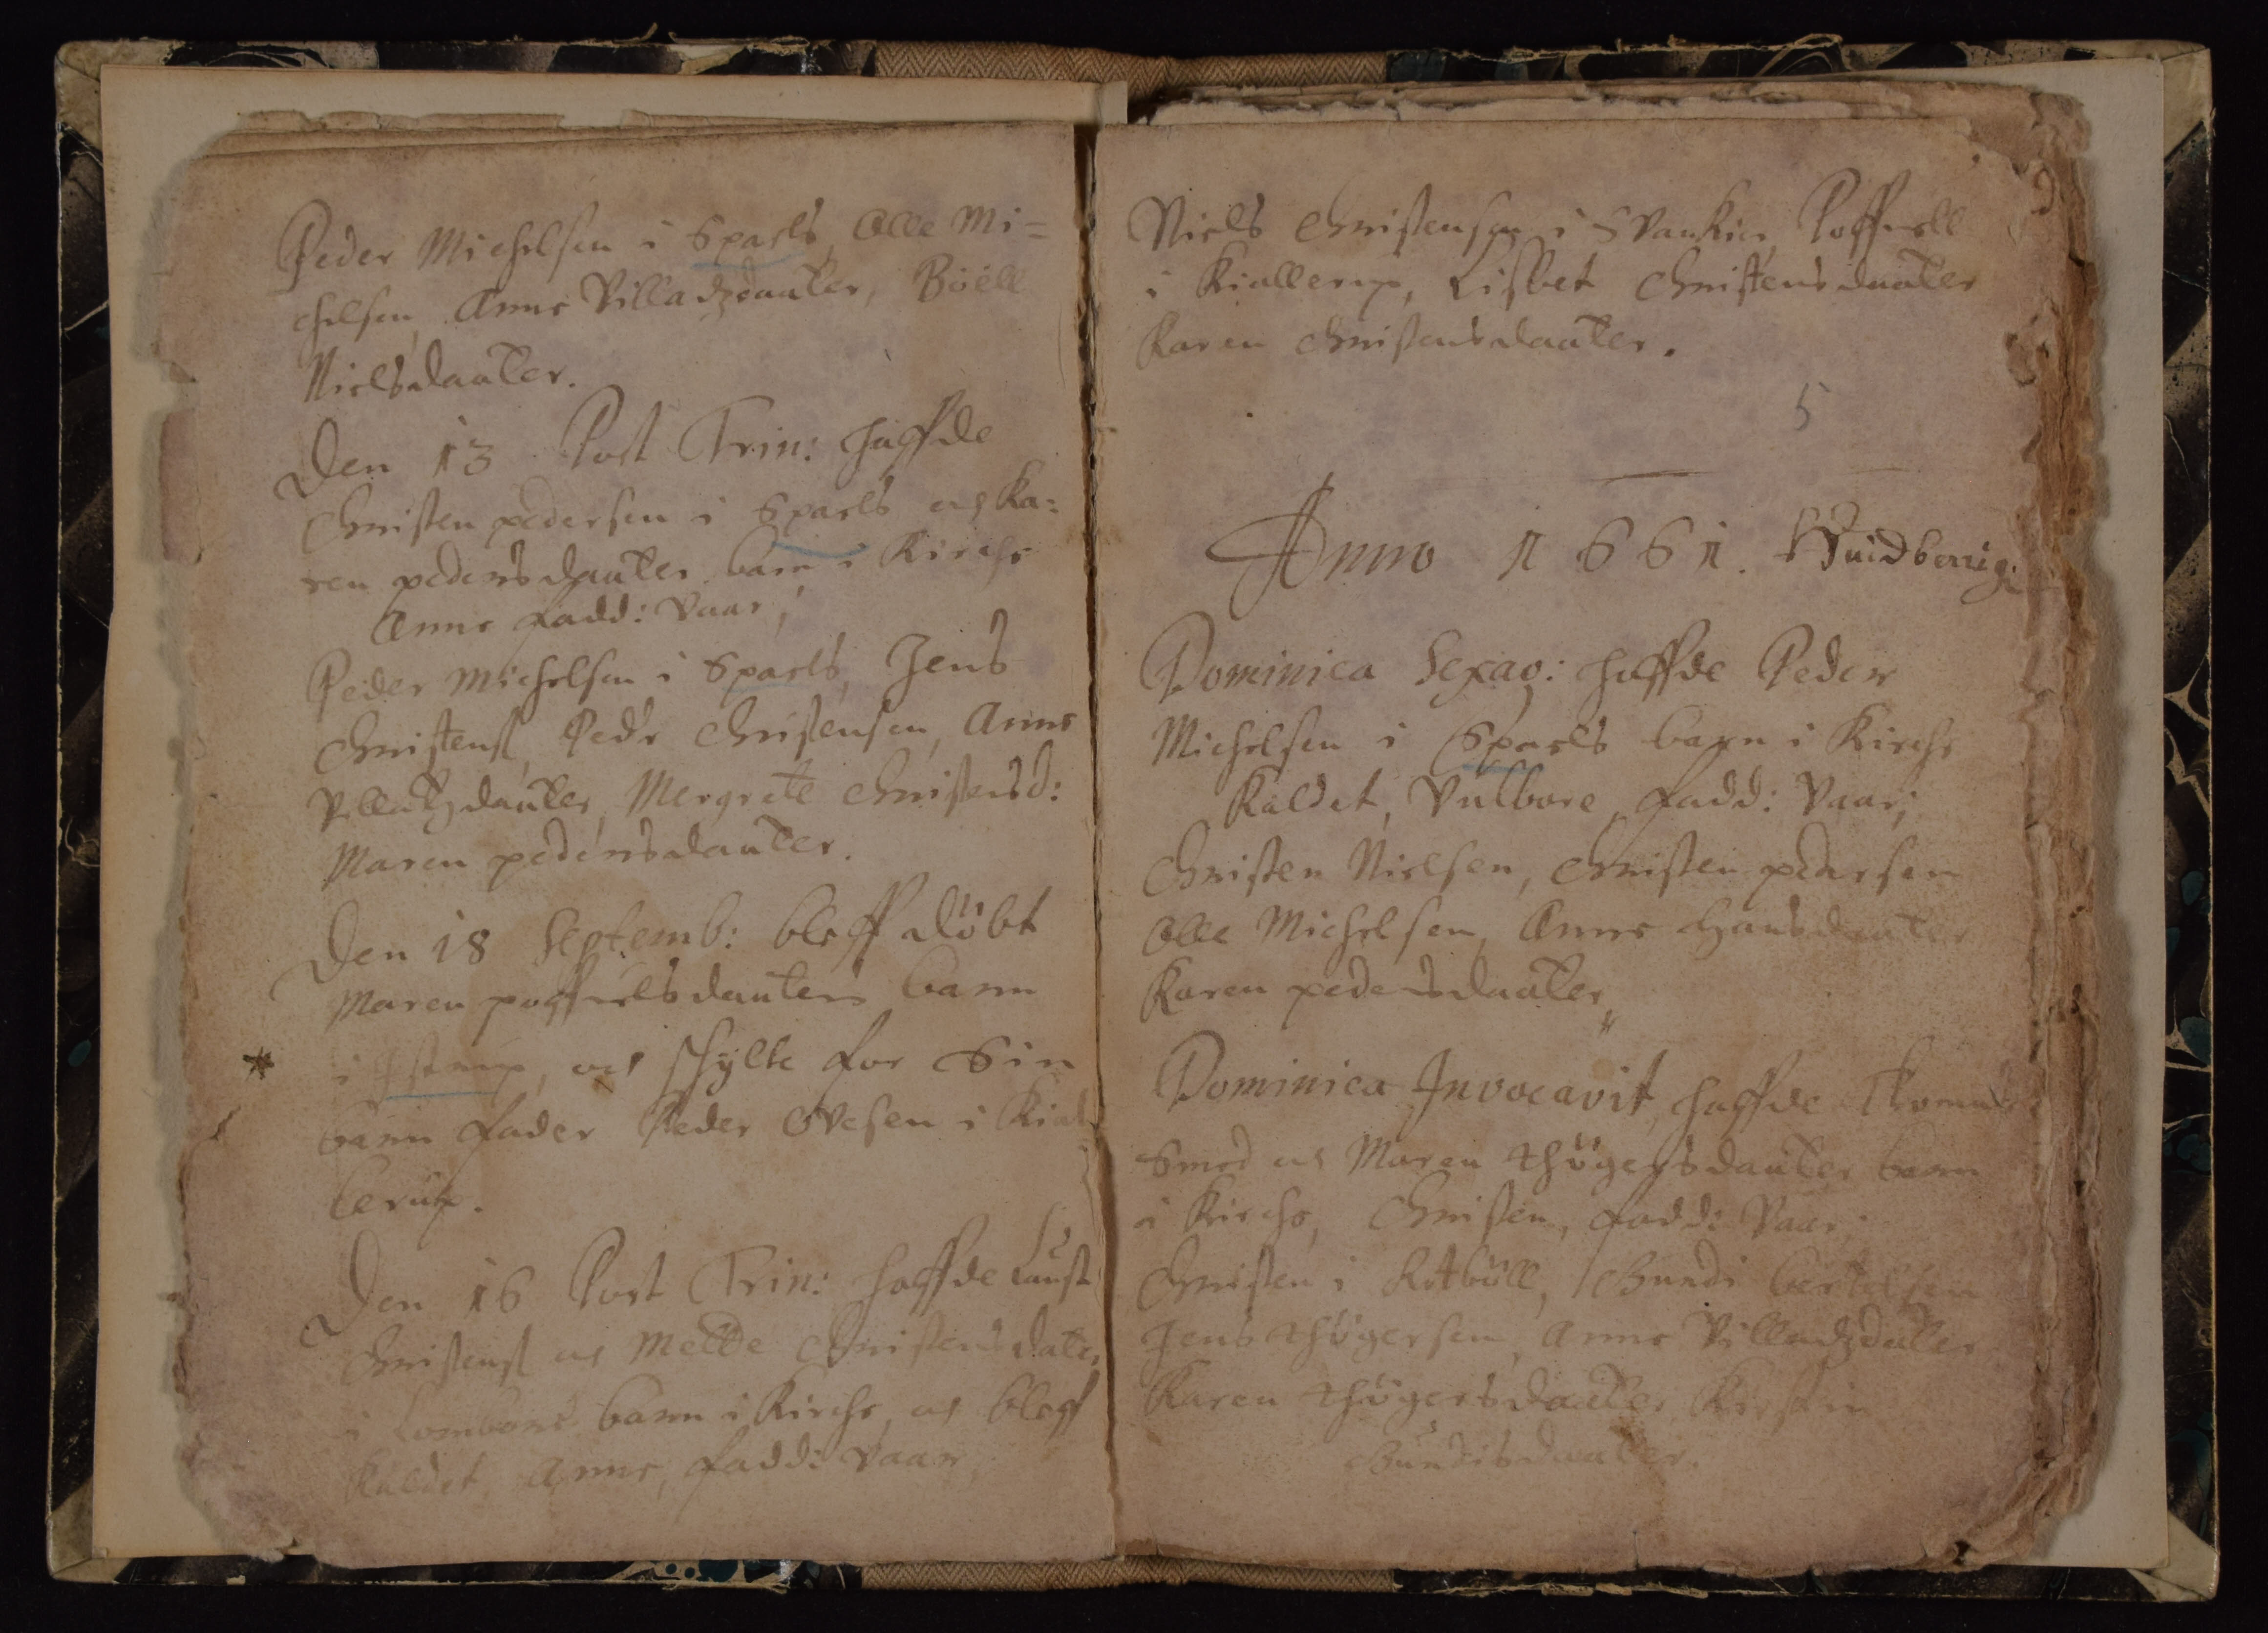Peder Michelsen i Sparls Olle Mi¬
cheilsen Anne Villadzdaater, Boell
Nielsdaater
Den 13 Post Trin: haffde
Christen Pedersøn i Sparls och Ka¬
ren Peders dauter barn Kirche
Anne Fadd: vaar
Peder Michelsen i Sparls, Jens
Christens Peder Christensen, Anne
Villindtzdautter Mergrete Christensd:
Maren pedersdauter.
Fen 18 Septemb: bleff døbt
Maren Poffuelsdauters barn
i Istrup, och skylte for sin
barn Fader Peder Ovesen i Kial¬
lerup.
Den: 16 Post Thrin: Haffde Laust
Christens och Mette Christensdater
i Lombons barn i Kirche, och bleff
kuldet Anne Fadd: vaar
Niels Christensen i Svankier Poffuell
i Kiallerup, Lisbet Christensdaatter
Karen Christentsdaater.
Anno 1661 Huiberrig
Dominica Sexag: Haffde Peder
Michelsen i Sparls barn i Kirche
kaldet Vulbore Fadd: vaar
Christen Nielsen, Christen Pedersen
Olle Michelsen, Anne Hansdauter
Karen pedersdaater
Dominica Invocavit, haffde Thomas
Smed och Maren Thørgersdaatter barn
i Kirche Christen. Fadd: vaar
Christen i Rotbøll; Gundi bertelsen
Jens Thøgersen Anne Villadzdater
Karen Thøgersdaater Khristin
Gundisdaater
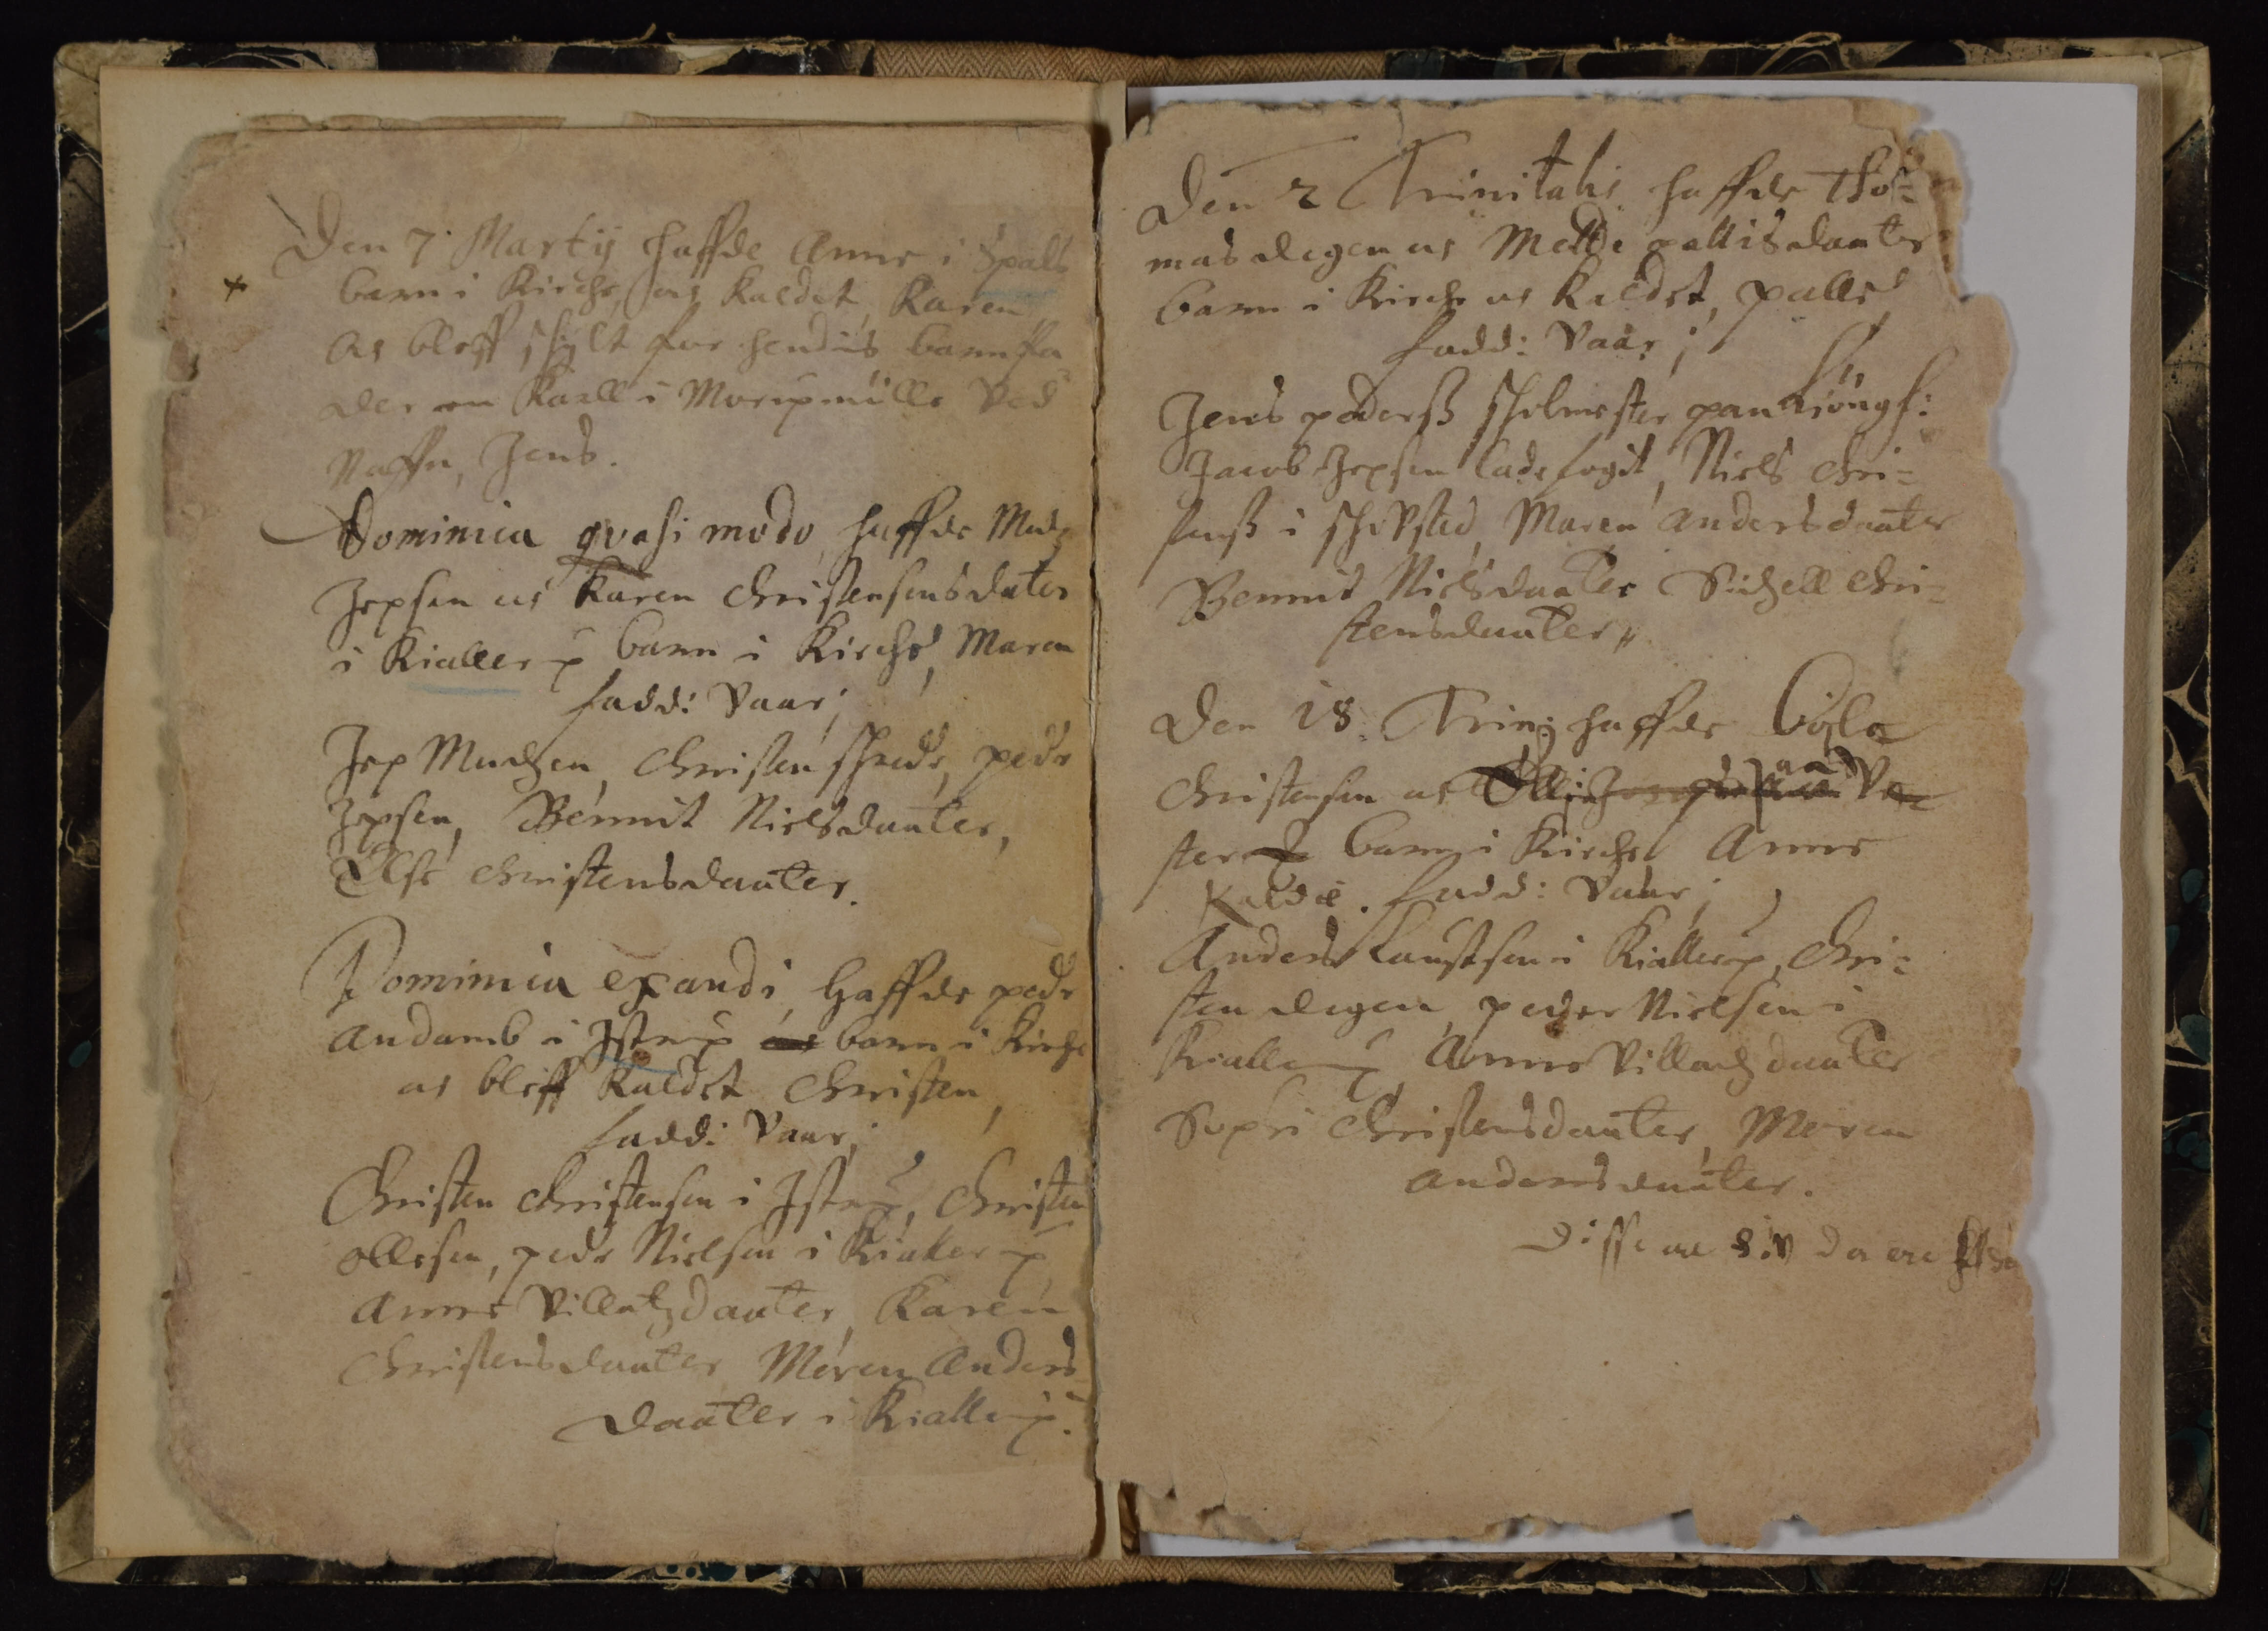Den 7 Martij haffde Anne i Spals
barn Kirche, och kaldet Karen
och bleff skylt for hendis barne Fa¬
der en Karll i Morupmølle ved
Naffn Jens.
Dominica Qvasi modo haffde Matz
Jepsen och Karen Christensensdater
i Kiallerup barn i Kirche, Maren
fadd: vaar
Jep Madsen, Christen sh##de pedr
Jepsen, Bennit Nielsdauter
Else Christensdauter
Dominica exaudi, haffde pode
Andamb i Istrup oc barn i Kirche
och bleff kaldet Christen
fadd: vaar
Christen Christensen i Istrup, Christn
Ollesen, pedr Nielsøn i Kiallerup
Anne Villatzdauter Karen
Christensdautter Maren Anders
daater i Kiallerup.
Den 2 Trinitatis. haffde Tho¬
mas degen och Mette pallisdaater
barn i Kirche oc kaldet palle
fadd: vaar;
Jens pederss Sholmester paa Liøngh:
Jacob Jepsen ladefogit Niels Cri¬
stenss i skovsted, Maren Andersdaater
Bennit Nielsdaater Sidzell Chri¬
stensdaater.
Den 18 Triny haffde O##le
Christensen och ##
## barn Kirche Anne
kaldet. fadd: vaar;
Anders Laustsen i Kiallerup Chri¬
sten degen peder Nielsen i
Kiallerup Anne Villadzdaater.
Sophi Christensdaater Maren
Andersdaatter
disse ere ## da ere ##